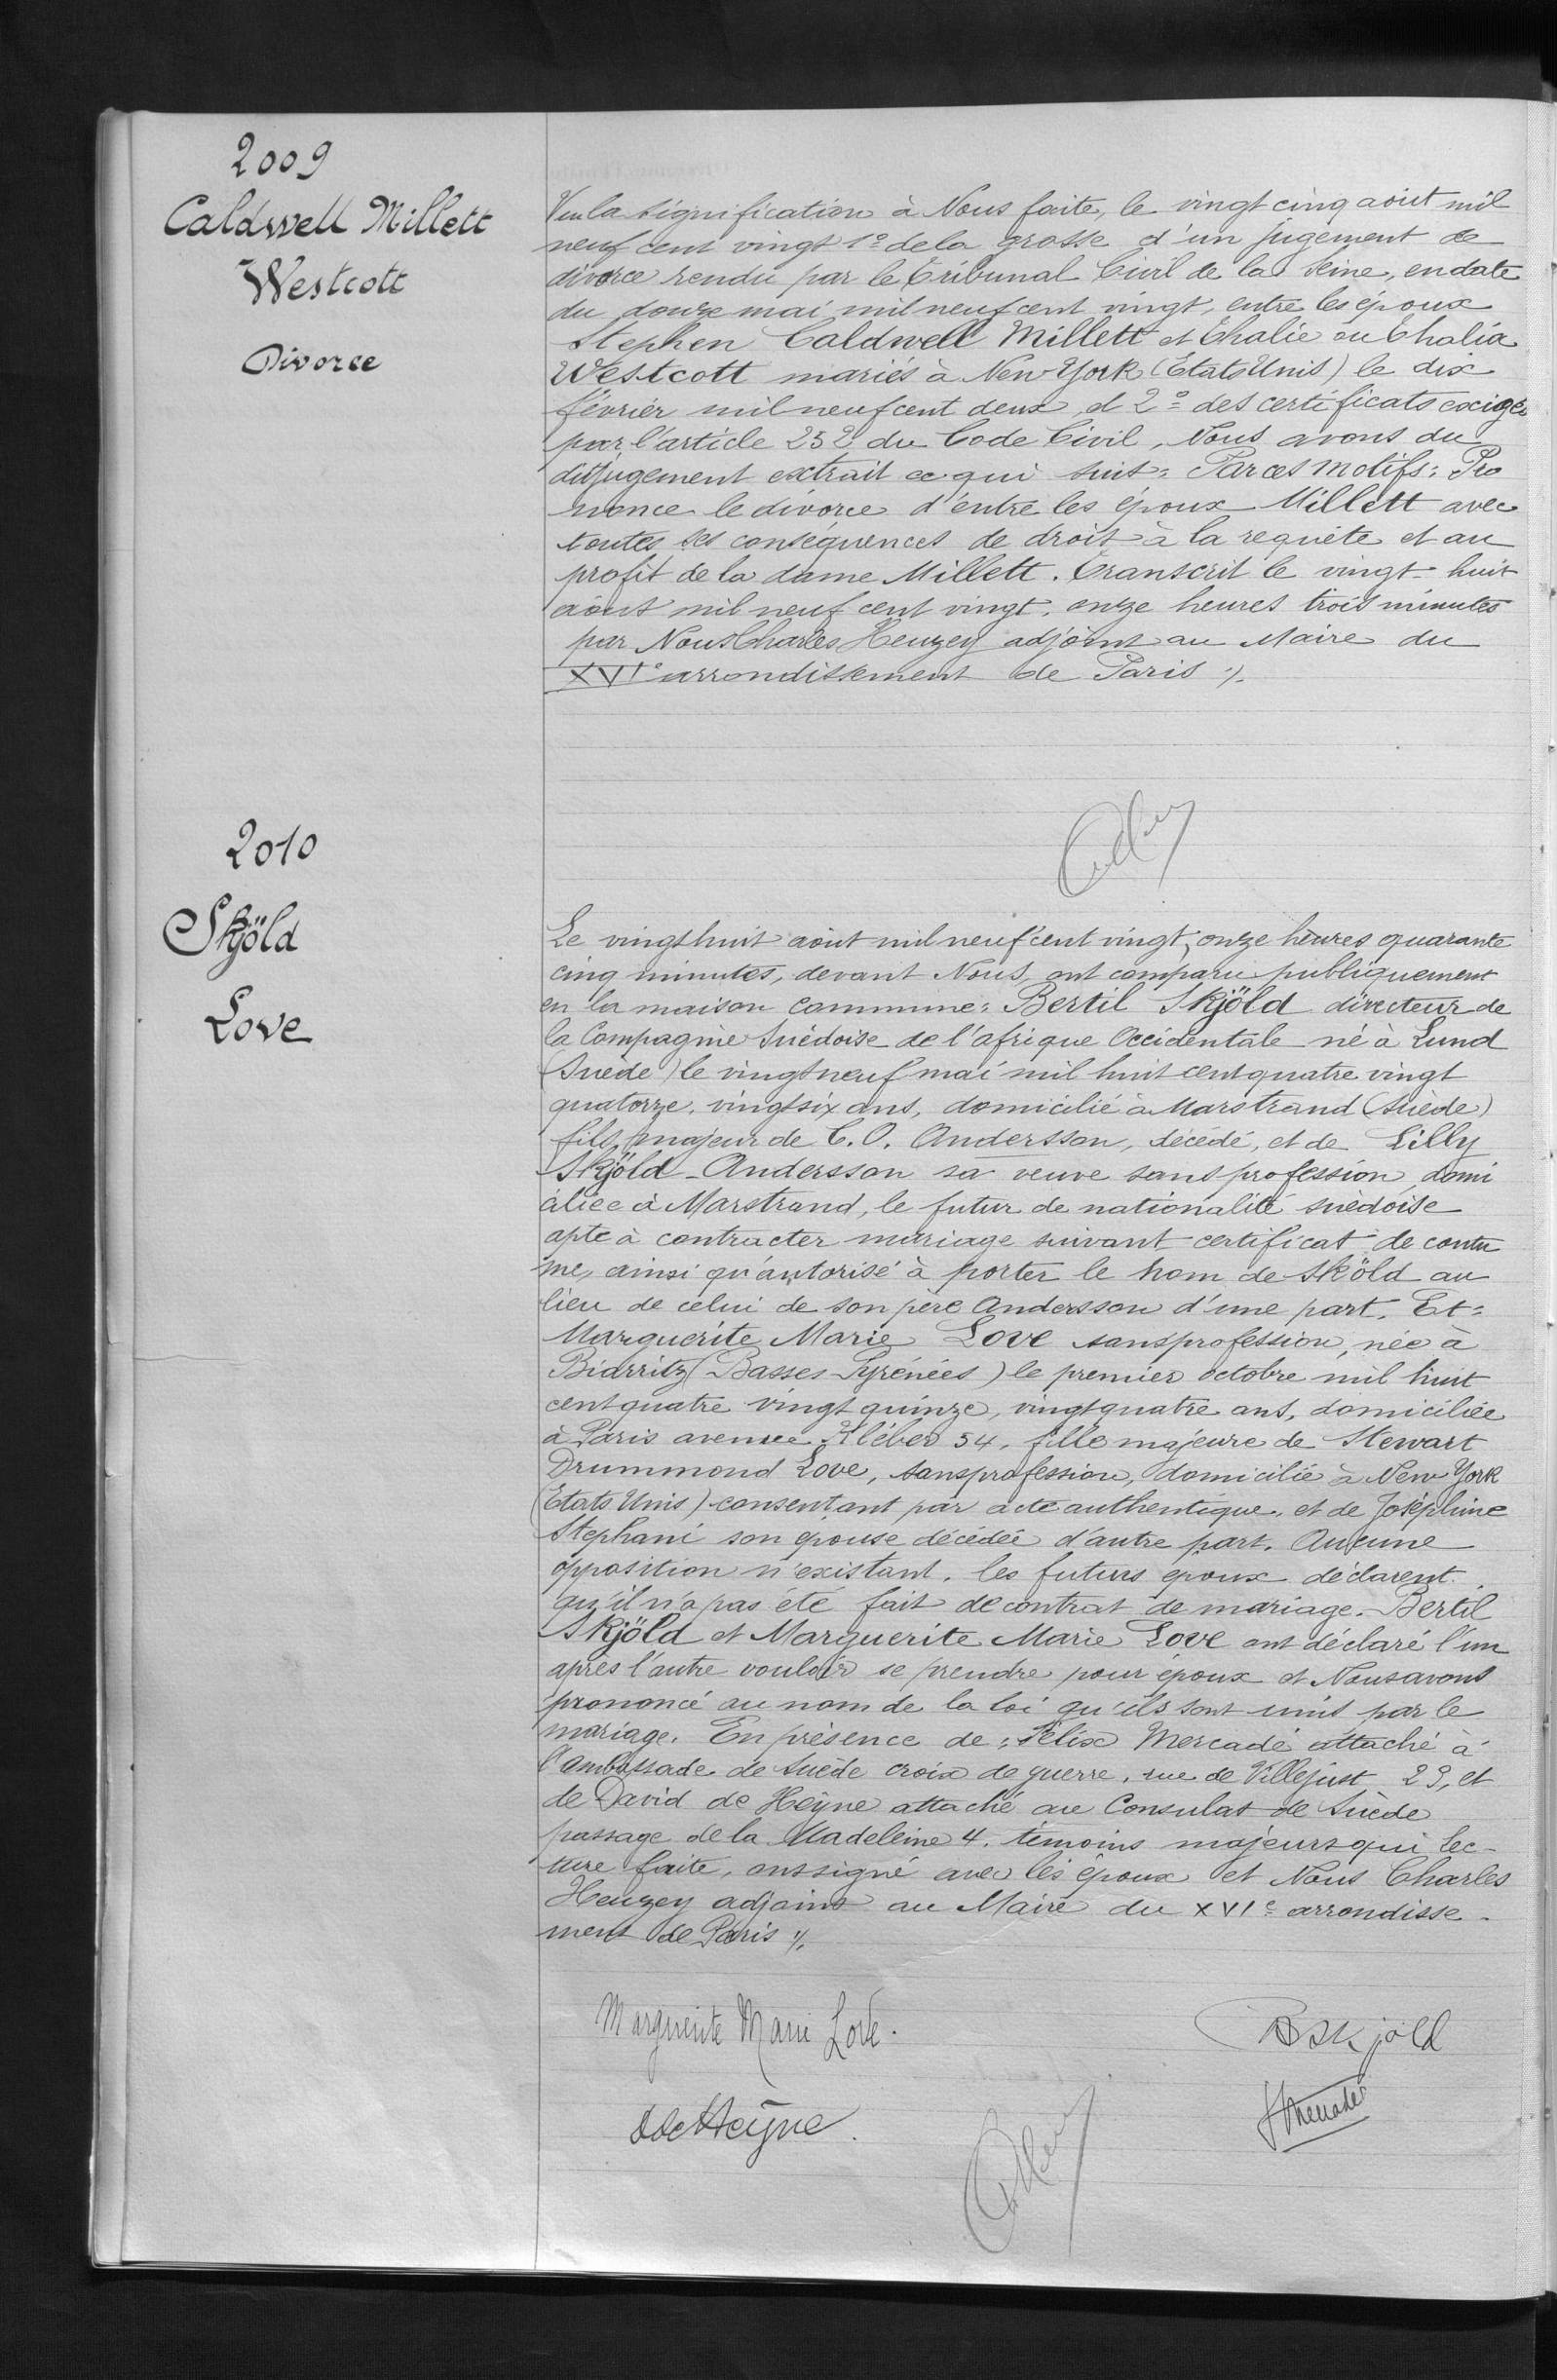2009
Caldwell Millett
Westcott
Divorce
Vu la signification à Nous faite le vingt-cinq août mil
neuf cent vingt 1° de la grosse d'un jugement de
divorce rendu par le Tribunal Civil de la Seine, en date
du douze mai mil neuf cent vingt entre les époux
Stephen Coldwell Millett et Chalie ou Chalia
Westcott mariés à New-York (Etats Unis) le dix
février mil neuf cent deux et 2° des certificats exigés
par l'article 252 du Code Civil, Nous avons du
ditjugement extrait ce qui suit: Par ces motifs: Pro
nonce le divorce d'entre les époux Millett avec
toutes ses conséquences de droit à la requéte et au
profit de la dame Millett. transcrit le vingt huit
aout mil neuf cent vingt: onze heures trois minutes
par Nous Charle Heuzey adjoint au Maire du
XVI arrondissement de Paris./.
2010
Shyöld
Love
Le vingt hui août mil neuf cent vingt, onze heures quarante
cinq minutes, devant Nous ont comparu publiquement
en la maison commune: Bertil Igöld directeur de
la Compagnie Suédoise de l'afrique Occidentale né à Lund
(Suède) le vingt neuf mai mil huit cent quatre vingt
quatorze. vingtsix ans, domicilié à Mastrand (Suède)
fils majeur de C.O. Andersson, décédé, et de Lilly
Sköld -Andersson sa veuve sans profession, domi
ciliée à Marstrand, le futur de nationalité suèdoise
apté à contracter mariage suivant certificat de coutu
me, ainsi qu'autorisé à porter le nom de Sköld au
lieu de celui de sn père Andersson d'une part. Et:
Marguerite Marie Love sansprofession, née à
Biarritz (Basses-Pyrénées) le premier octobre mil huit
cent quatre vingt quinze vingt quatre ans, domiciliée
à Paris avenue Kléber 54, fille majeure de Stenvart
Drummond Love, sansprofession, domicilié à New-York
(Etats Unis) consentant par acte authentique et de Joséphine
Séphani son épous décédée d'autre part. Aucune
opposition n'existant. les futurs époux déclarent
qu'il n'a pas été fait de contrat de mariage. Bertil
Sköld et Marguerite Marie Love ont déclaré l'un
après l'autre vouloir se prendre pour époux et Nous avons
prononcé au nom de la loi qu'ils sont unis par le
mariage. En présence de: Felix Mercadé attaché à
l'Ambassade de Suède croix de guerre, rue de Villejust 23, et
de David de Hêyne attaché au Consulat de Suède
passage de la Madeleine 4 témoins majeurs qui lec-
ture faite, ont signé avec les époux et Nous Charles
Heuzey adjoint au Maire du XVI arrondisse-
ment de Paris./.
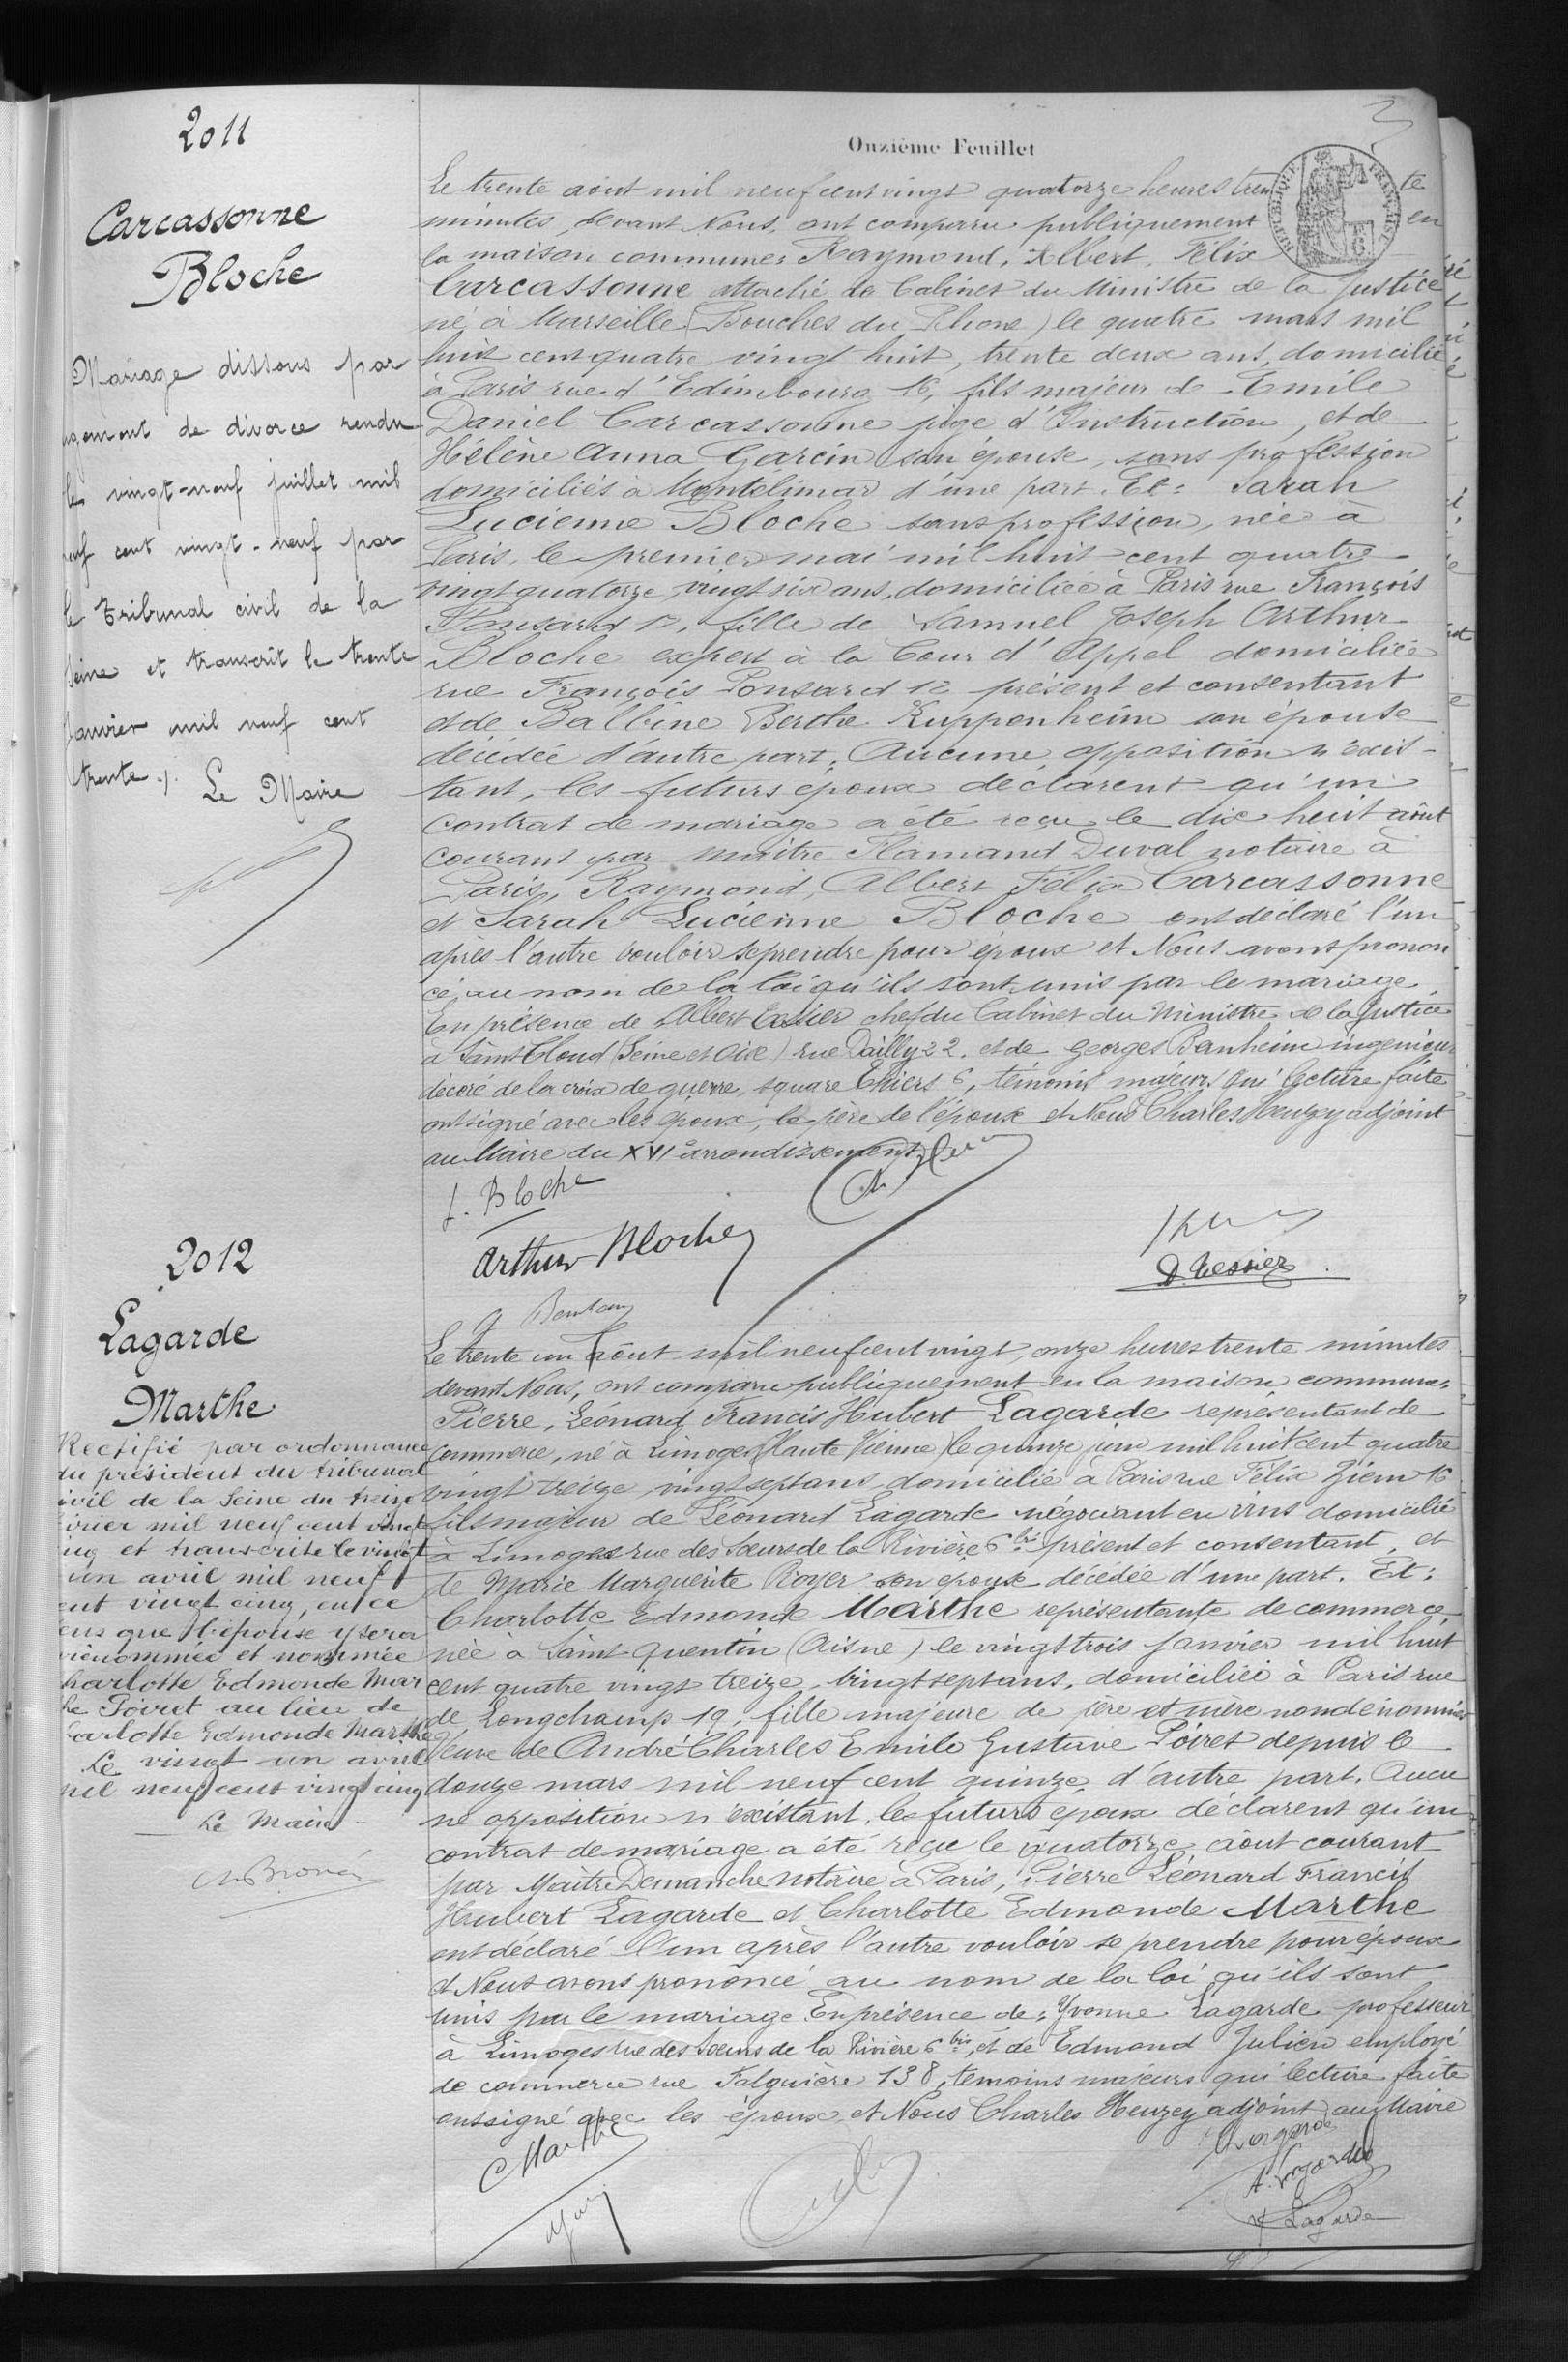2011
Carcassonne
Bloche
Le trente avril mil neuf cent vingt quatorze heures trente
minutes, devant Nous ont comparu publiquement en
la maison commune Raymond Albert, félic
Carcassonne, attaché de Cabinet du Ministre de la justice
né à Marseille (Bouches du Rhone) le quatre mars mil
huit cent quatre vingt huit, trente deux ans domicilié
à Paris rue d'Edimbourg 16, fils majeur de Emile
Daniel Carcassonne juge d'Instruction, et de
Hélène Anna Garcin, son épouse, sans profession,
domiciliés à Montélimar d'une part. Et: Sarah
Lucienne Bloche sans profession, née à
Paris, le premier mai mil huit cent quatre-
vingt quatorze vingt six ans, domiciliée à Paris rue François
Ponsard 12, fille de Samuel Joseph Arthur-
Bloche expert à la Cour d'Appel domicilié
rue François Poncard 12 présent et consentant
et de Balbine Berthe. Ruppenheim son épouse
décédé d'autre part; Aucune opposition n'éxis
tant, les futurs époux déclarent qu'un
contrat de mariage a été reçu le dix huit août
courant par maitre Flamand Duval notaire à
Paris, Raymond, Albert Félix Carcassonne
et Sarah Lucienne Bloche ont déclaré l'un
après l'autre vouloir se prender pour époux et Nous avons pronon
cé au nom de la Loi qu'ils sont unis par le mariage.
En présence de Albert Exssier chef du Cabinet du Ministre de la Justice
à Saint Cloud (Seine et Oise) rue Dailly 22, et de Georges Banhcine ingénieur
décoré de la croix de guerre, square Thiers 6, témoins majeurs qui lecture faite
ont signé avec les époux, le père de l'époux et Nous Charle Heuzey adjoint
au Maire du XVI° arrondissement.
2012
Lagarde
Marthe
Le trente un août mil neuf cent vingt, onze heures trente minutes
devant Nous, ont comparu publiquement en la maison commune
Pierre Léonard Francis Hubert Lagarde représentant de
commerce, né à Limoges (Haute Vienne) le quinze juin mil huit cent quatre
vingt seize, vingt sept ans domicilié à Paris rue Félix Ziem 16
fils majeur de Léonard Lagarde négociant en vins domicilié
à Limoges rue des Soeurs de la Rivière 6 bis présent et consentant et
de Marie Marguerite Royer son épouse décédée d'une part. Et:
Charlotte Edmonde Marthe représentante de commerce
née à Saint Quentin (Aisne) le vingt trois Janvier mil huit
cent quatre vingt treize vingt sept ans, domiciliée à Paris rue
de Longchamp 19, fille majeure de père et mère nomdénommés
Veyve de André Charles Emile Gustave Poiret depuis le
douze mars mil neuf cent quinze, d'autre part. Aucu-
ne opposition n'existante, les futurs époux déclarent qu'un
contrat de mariage a été reçu le quatorze; août courant
par Maitre Demanche notaire à Paris, Pierre Léonard Francis
Huchert Lagarde et Charlotte Edmonde Marthe
ont déclaré l'un après l'autre vouloir se prendre pour époux
et Nous avons prononcé au nom de la loi qu'ils sont
unis par le mariage. En présence de: Yvonne Lagarde, professeur
à Limoges rue des Soeurs de la Rivière 6 bis, et de Edmond Julien employé
de commerce rue Falguière 138, témoins majeurs qui lecture faite
ontsigné avec les époux et Nous Charles Heuzey adjoint au Maire